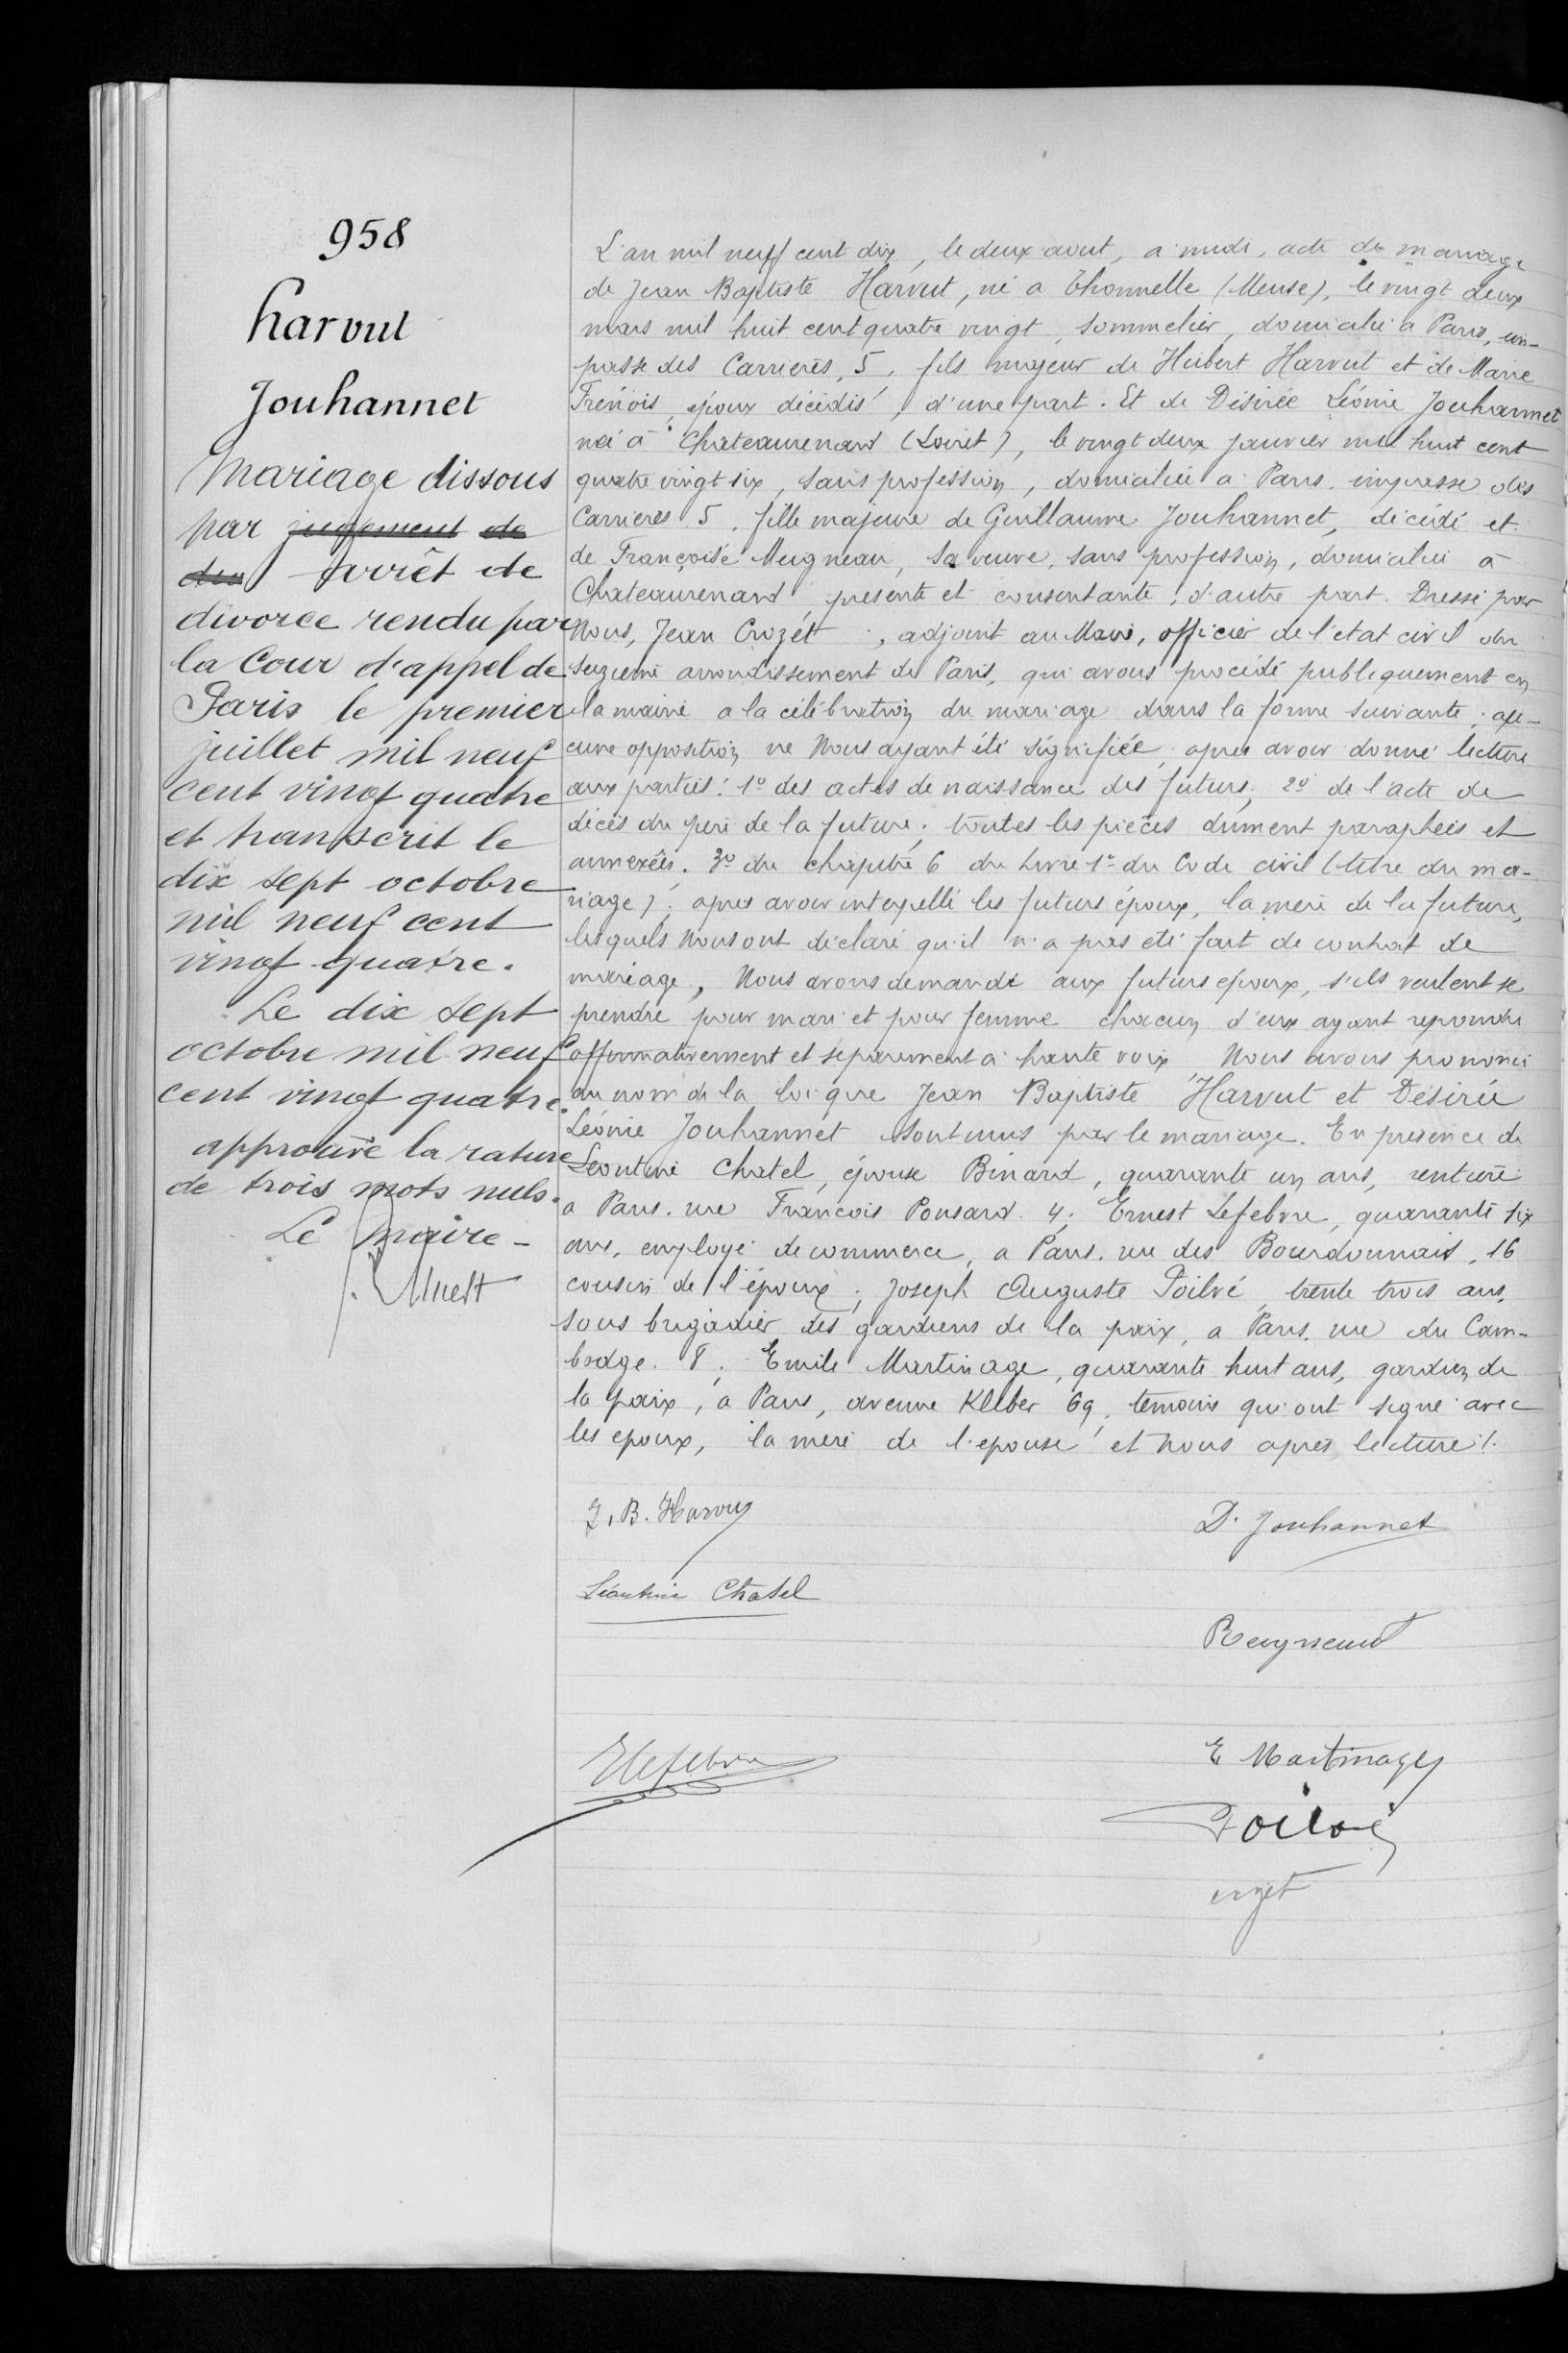958
Harvut
Jouhannet
L'an mil neuf cent dix, le deux aout, a midi acte de mariage
de Jean Baptiste Harvut, né à Thonnelle (Meuse), le vingt deux
mars mil huit cent quatre vingt, sommelier, domicilié a Paris, im-
passe des Carrières, 5, fils majeur de Hubert Harvut et de Marie
Frénois, époux décédés, d'une part. Et de Désirée Léonie Jouhannet
née à Chateaurenard (Loiret), le vingt deux janvier mil huit cent
quatre vingt-six, sans profession, domiciliée à Paris, impasse des
Carrieres 5, fille majeure de Guillaume Jouhannet, décédé et
de Françoise Meigneau, sa veuve, sans profession, domiciliée à
Chateaurenard présente et consentantee, d'autre part. Dressé par
Nous, Jean Crozet, adjoint au Maire, officier de l'état civil du
seizième arrondissement de Paris, qui avons procédé publiquement en
la mairie a la célébration du mariage sous la forme suivante: au-
cune opposition ne Nous ayant été signifié après avoir donné lecture
aux parties: 1° des actes de naissance des futurs, 2° de l'acte de
décès du père de la future; toutes les pièces dument paraphées et
annexés. 3° du chapitre 6 du Livre 1° du Code civil (titre du mar-
iage) après avoir interpellé les futurs époux, la mère de la future
lesquels Nous ont déclaré qu'il n'a pas été fait de contrat de
mariage, Nous avons demandé aux futurs époux, s'ils veulent se
prendre pour mari et pour femme chacun d'eux ayant répondu
affirmativement et séparément à haute voix Nous avons prononcé
au nom de la loi que Jean Baptiste Harvut et Désirée
Léonie Jouhannet sont unis par le mariage. En présence de
Léontine Chatel, épouse Binard, quarante un ans, rentière
à Paris rue François Ponsard 4; Ernest Lefebvre, quarante six
ans, employé de commerce a Paris, rue des Bourdonnais, 16
cousin de l'époux; Joseph Auguste Poilvé, trente trois ans,
sous brigadier des gardiens de la paix, a Paris rue du Cam-
bodge 8. Emile Martinage quarante huit ans, gardien de
la paix à Paris avenue Kleber 69; témoins qui ont signé avec
les époux, la mère de l'épouse et Nous après lecture ./.
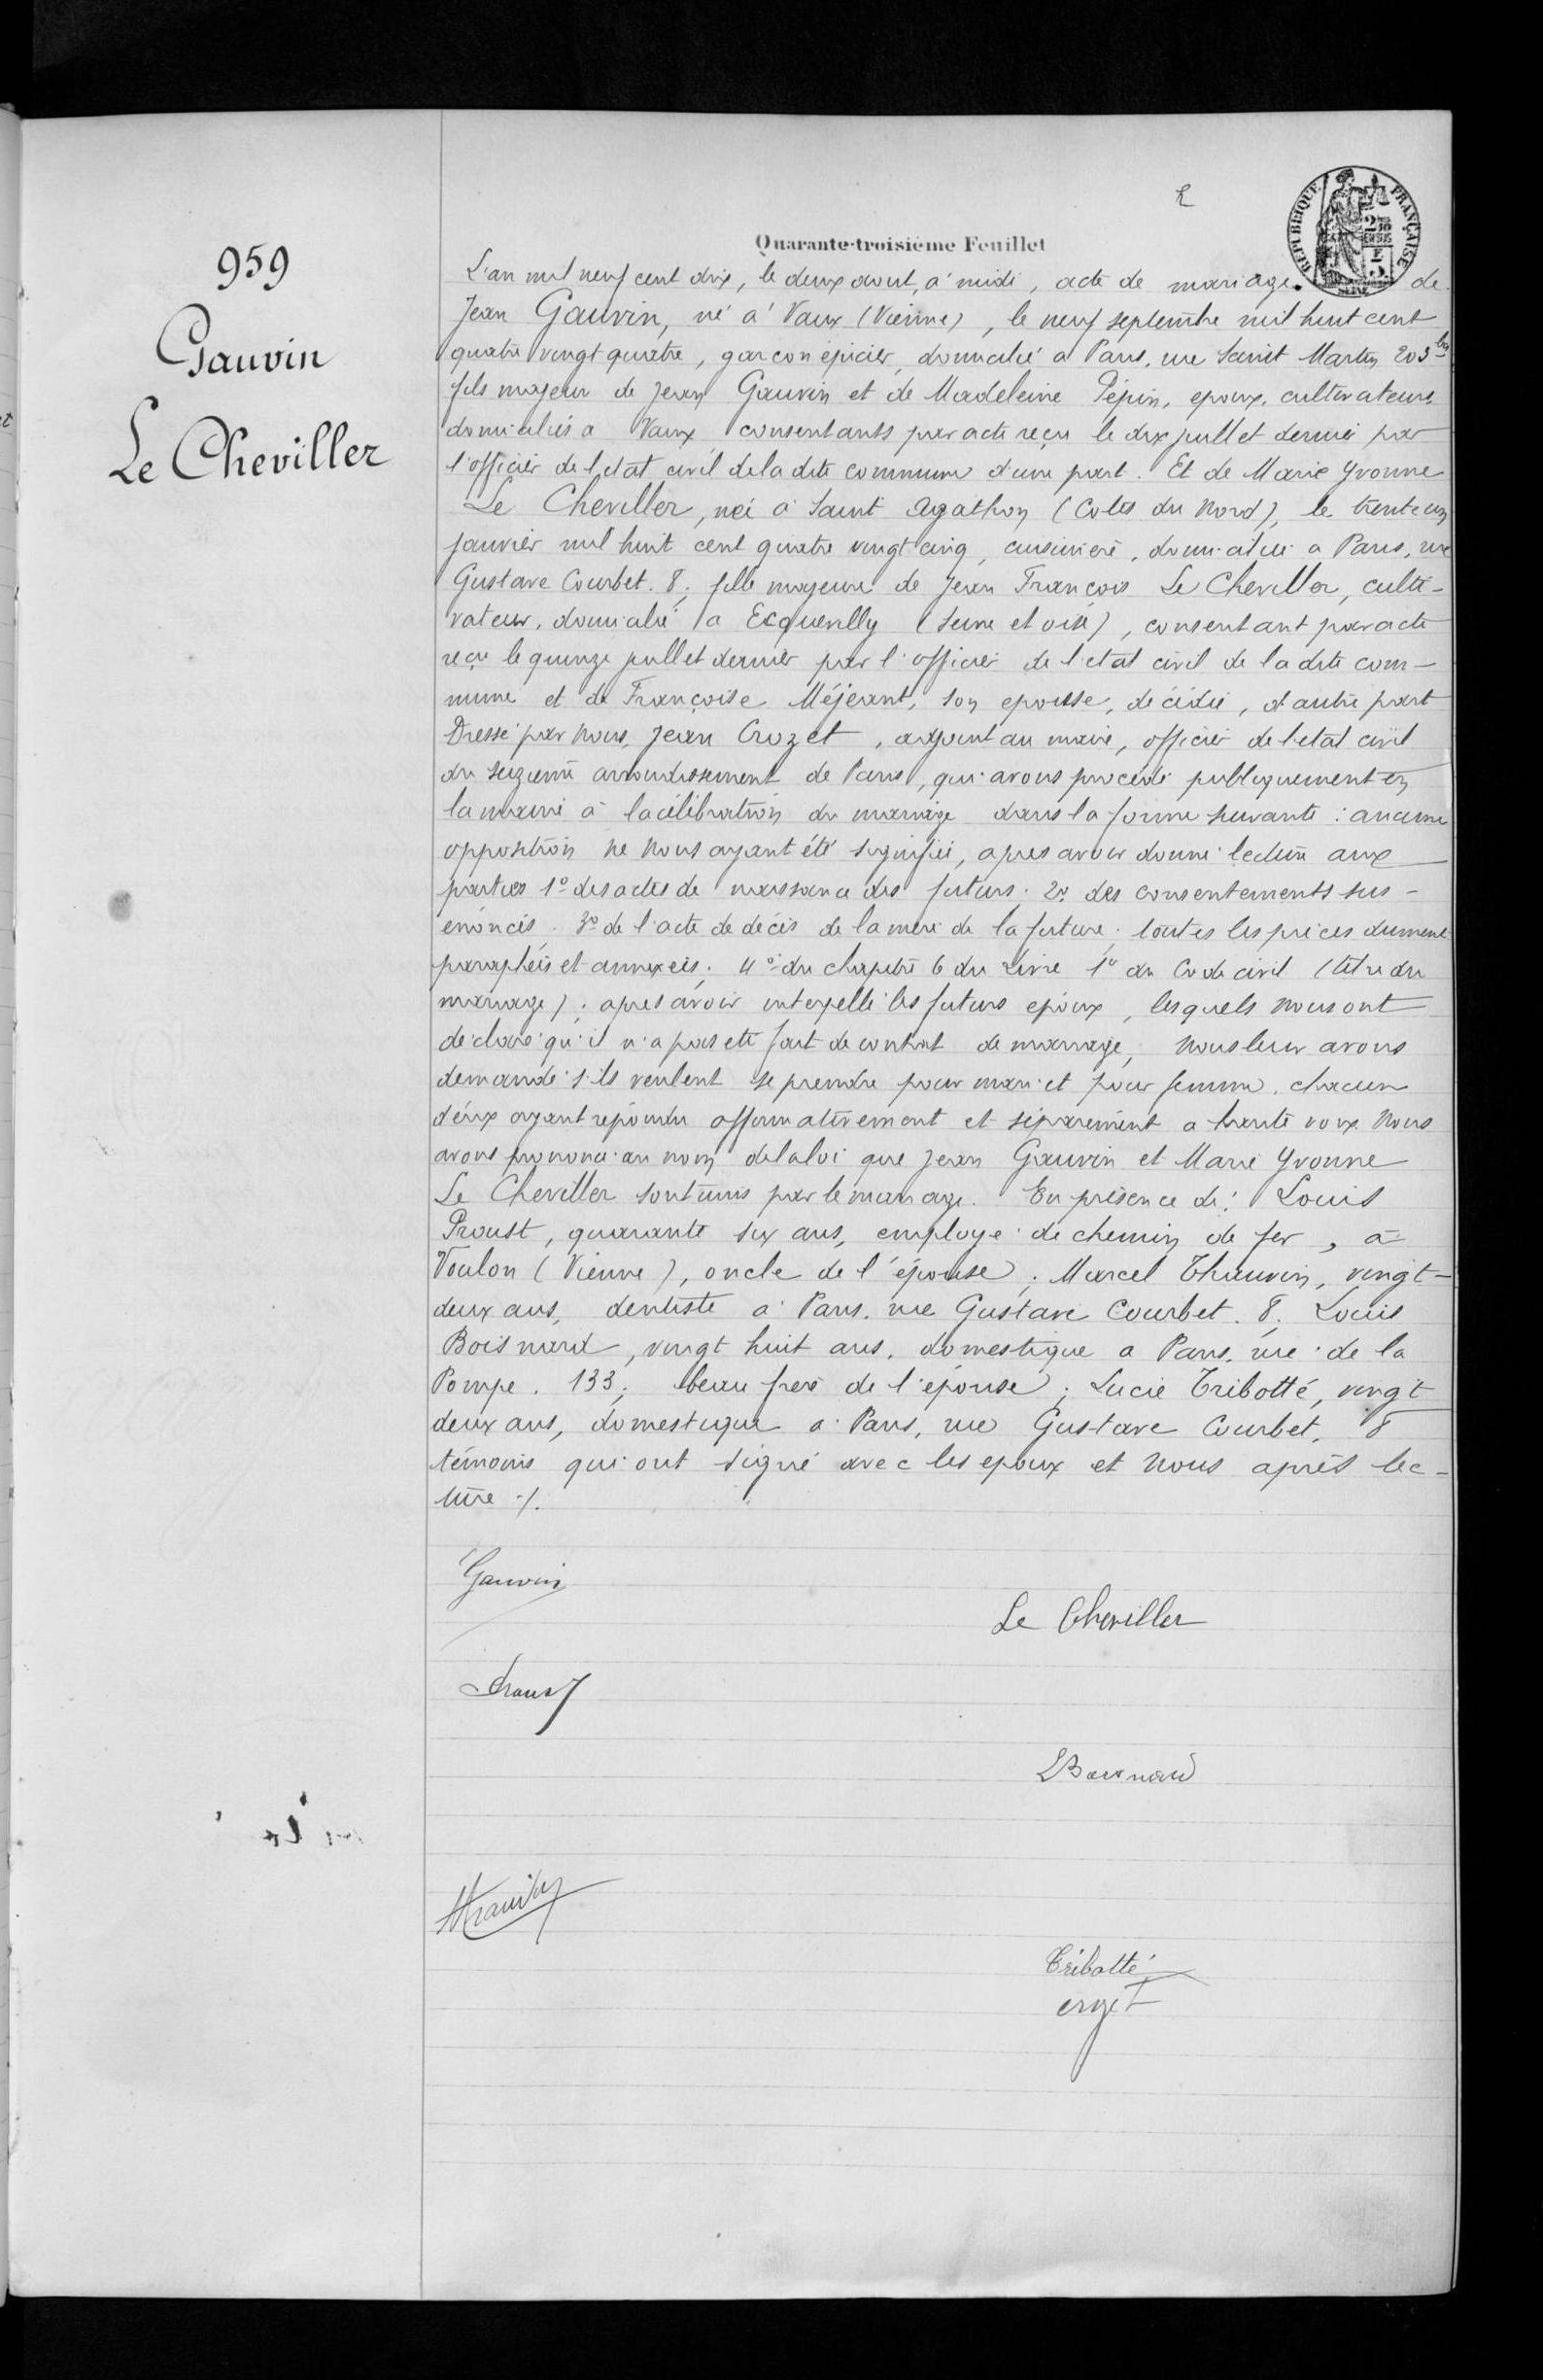959
Gauvin
Le Cheviller
L'an mil neuf cent dix, le deux aout, à midi, acte de mariage de
Jean Gauvin, né à Vaux (Vienne), le neuf septembre mil huit cent
quatre vingt quatre, garçon épicier, domicilié à Paris, rue Saint Martin 203 bis
fils majeur de Jean Gauvin et de Madeleine Pépin, epoux, cultivateurs,
domiciliés à Vaux consentants par acte reçu le dix juillet dernier par
l'officier de l'etat civil dela dite commune d'une part. Et de Marie Yvonne
Le Cheviller, née à Saint Agathon (Cotes du Nord), le trente un
janvier mil huit cent quatre vingt cinq, cuisinière, domiciliée à Paris, rue
Gustave Courbet 8, fille majeure de Jean François Le Chevillon, culti-
vateur, domicilié a Ecquevilly (Seine et Oise), consentant par acte
reçu le quinze juillet dernier par l'officier de l'tat civil de ladite com-
mune et de Françoise Méjeant, son épouse, décédés, d'autre part
Dressé par Nous, Jean Crozet, adjoint au maire, officier de l'etat civil
du seizième arrondissement de Paris, qui avons procédé publiquement en
la mairie à la célébration du mariage dans la forme suivante: aucune
opposition ne nous ayant été signifié, après avoir donné lecture aux
parties 1° des actes de naissances des futurs 2° des consentements sus-
énoncés; 3° de l'acte de décès de la mère de la future; toutes les pièces dument
paraphées et annexées; 4° du chapitre 6 du Livre 1er du Code civil (titre du
mariage): après avoir interpellé les futurs époux, lesquels nous ont
déclaré qu'il n'a pas été fait de contrat de mariage, nous leur avons
demandé s'ils veulent se prendre pour mari et pour femme chacun
d'eux ayant répondu affirmativement et séparément à haute voix Nous
avons prononcé au nom de la loi que Jean Gauvin et Marie Yvonne
Le Cheviller sont unis par le mariage. En présence de: Louis
Proust, quarante six ans, employé de chemin de fer, à
Voulon (Vienne), oncle de l'épouse; Marcel Thuvin, vingt-
deux ans, dentiste à Paris, rue Gustave Courbet, 8; Louis
Boisnard, vingt huit ans, domestique a Paris rue de la
Pompe, 133; beau frère de l'épouse; Lucie Triotté, vingt
deux ans, domestique a Pau, rue Gustave Courbet, 8
témoins qu'ont signé avec les epoux et nous après lec-
ture ./.